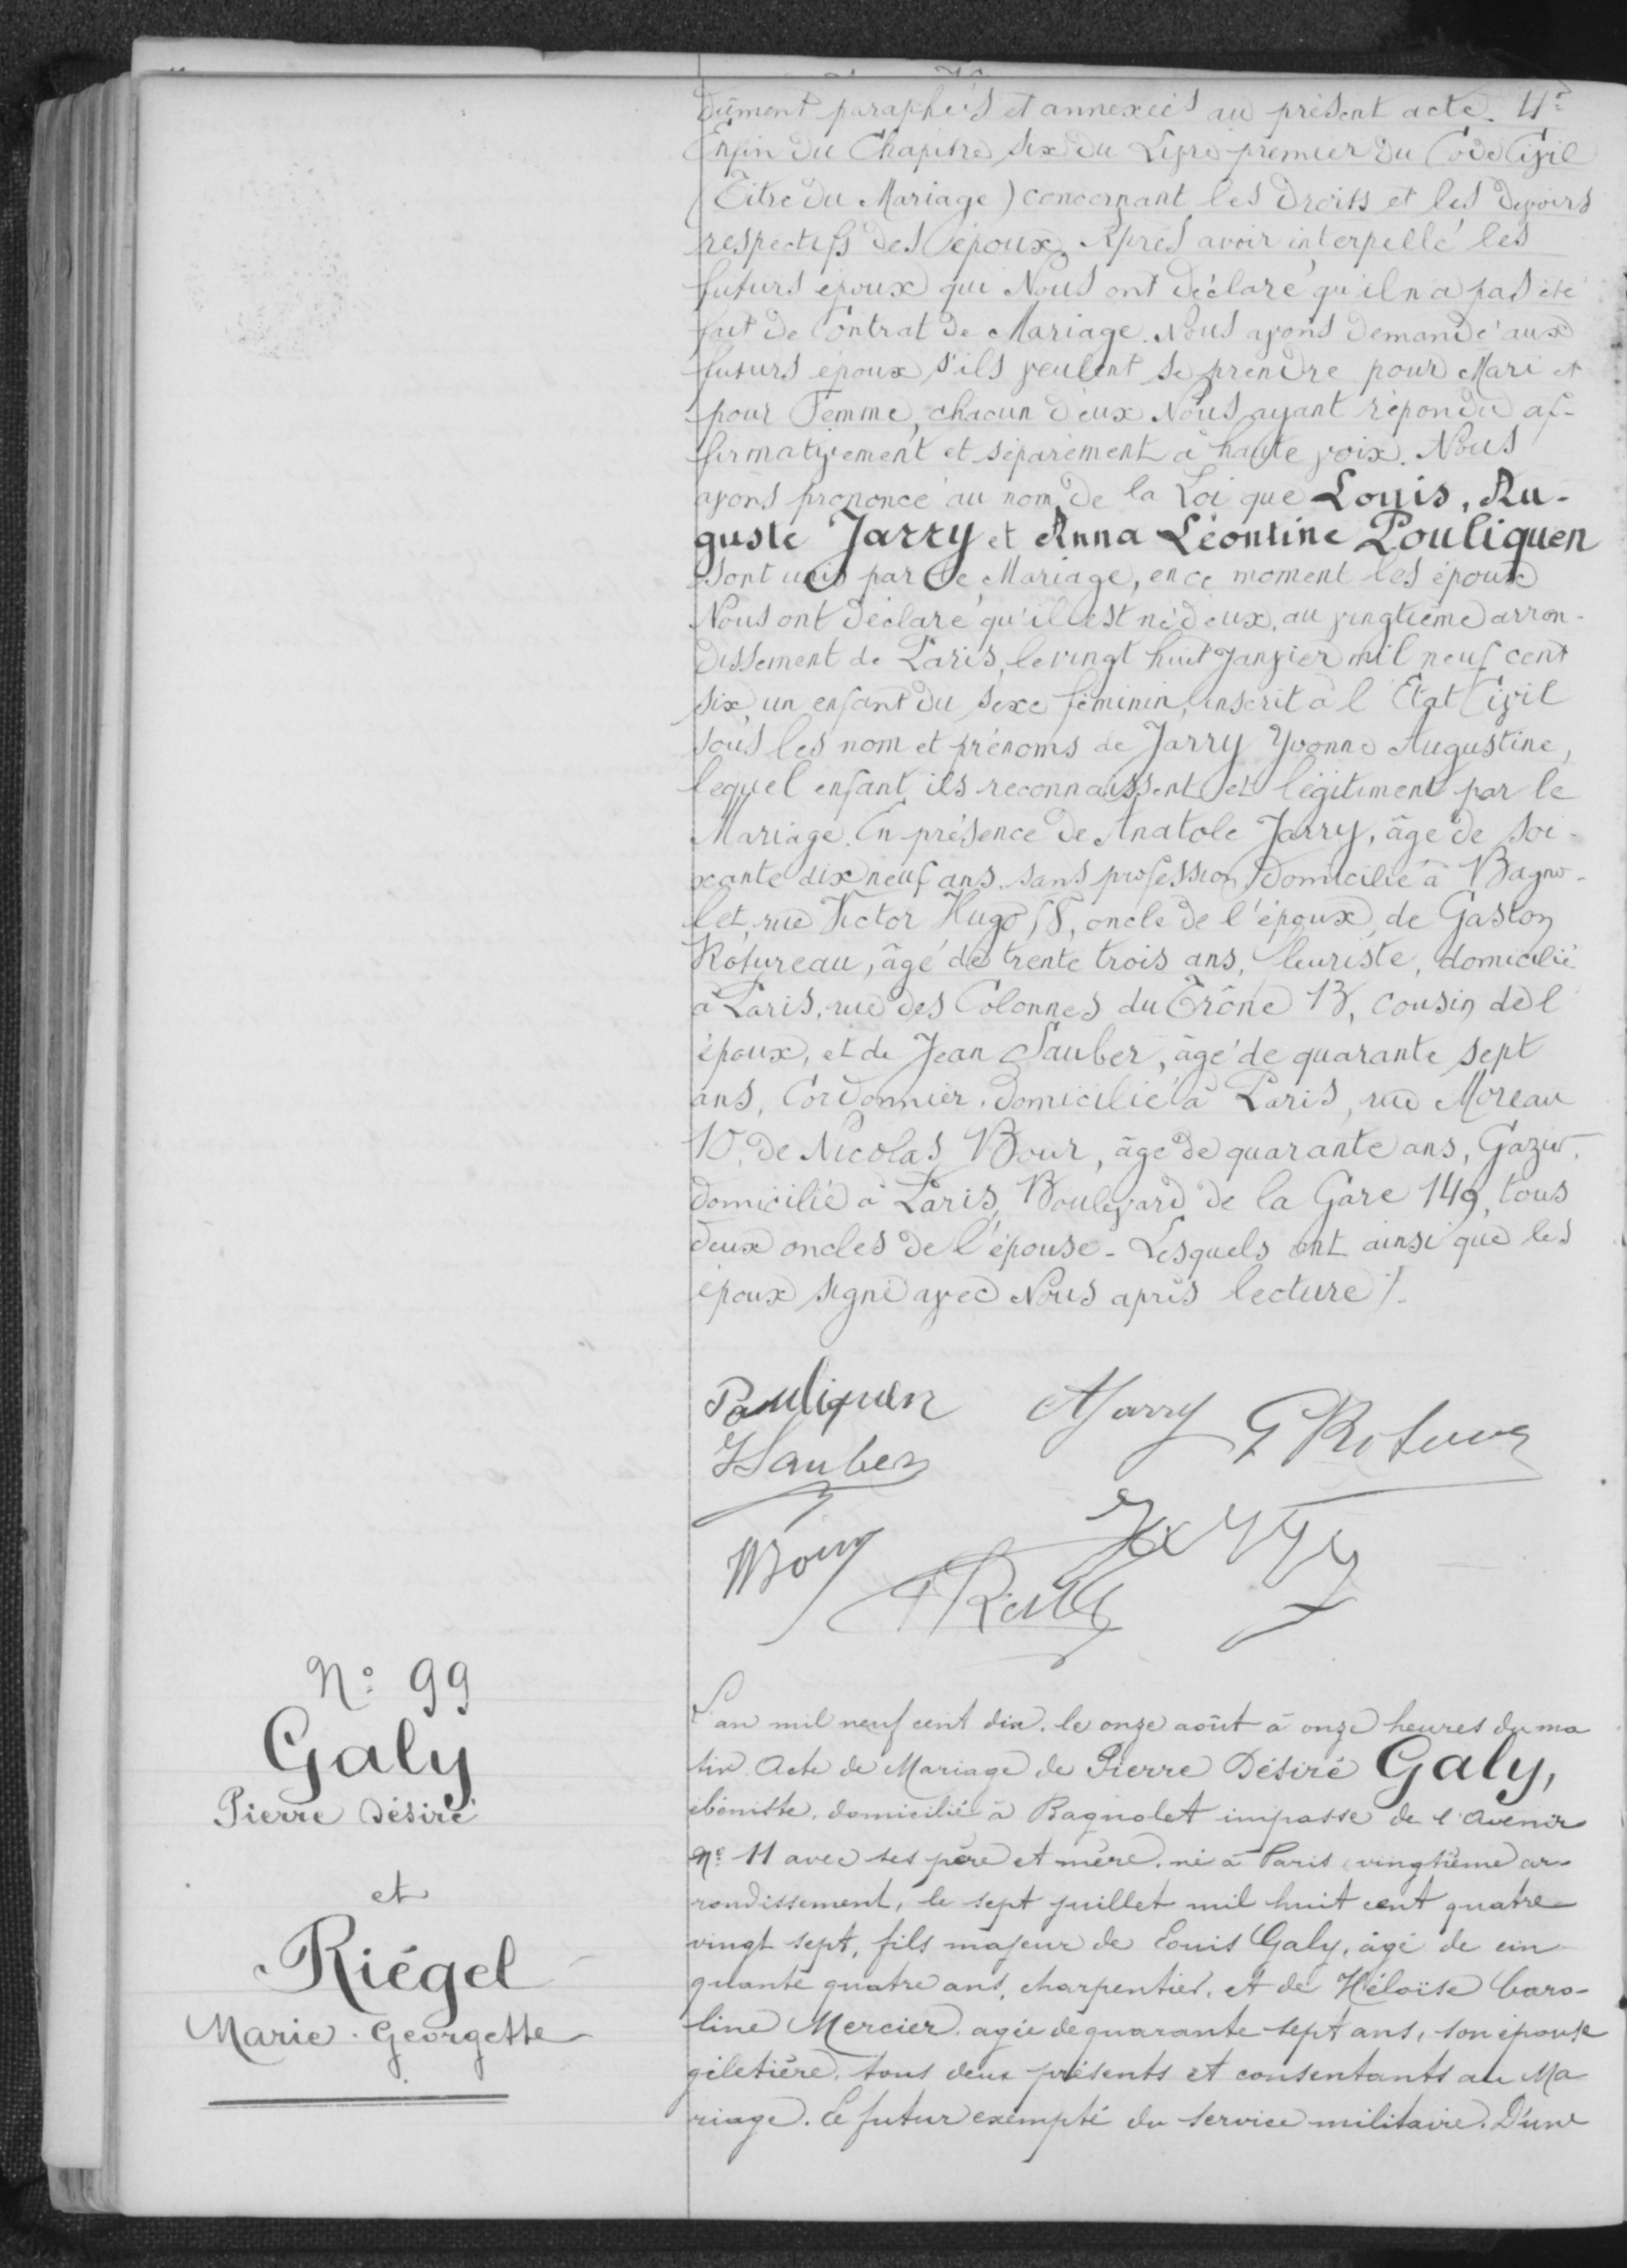dûment paraphées et annexées au présent acte. 4°
Enfin du Chapitre six du Livre premier du Code Civil
(Titre du Mariage) concernant les Droits et les devoirs
respectifs des époux. Après avoir interpellé les
futurs époux qui Nous ont déclaré qu'il n'a pas été
fait de contrat de Mariage. Nous ayons demandé aux
futurs époux s'ils veulent se prendre pour Mari et
pour Femme, chacun d'eux. Nous ayant répondu af-
firmativement et séparément à haute voix. Nous
avons prononcé au nom de la Loi que Louis, Au-
guste Jarry et Anna Léontine Pouliquen
sept unis par le Mariage, en ce moment les époux
Nous ont déclaré qu'il est né deux, au vingtième arron-
dissement de Paris, le vingt huit janvier mil neuf cent
six, un enfant du sexe féminin, inscrit à l'Etat Civil
sous les noms et prénoms de Jarry Yvonne Augustine,
lequel enfant ils reconnaissent et légitiment par le
Mariage. En présence de Anatole Jarry, âgé de soi
xante dix neuf ans sans profession domicilié à Bagno-
let, rue Victor Hugo 58, oncle de l'époux de Gaston
Rotureau, âgé de trente trois ans, fleuriste, domicilié
à Paris, rue des Colonnes du Trône 13, cousin de l'
époux, et de Jean Sauber, âgé de quarante sept
ans, cordonnier, domicilié à Paris, rue Morea
15 de Nicolas Bour, âgé de quarante ans, Gazur,
domicilié à Paris, Boulevard de la Gare 149, tous
deux oncles de l'épouse. Lesquels ont ainsi que les
époux signé avec Nous après lecture./.
N°99
Galy
Pierre Désiré
et
Riégel
Marie Georgette
L'an mil neuf cent dix le onze août à onze heures du ma
tin acte de Mariage, de Pierre Désiré Galy,
ébéniste, domicilié à Bagnolet impasse de l'avenir
N°:11 avec ses père et mère, né à Paris, vingtième ar-
rondissement, le sept juillet mil huit cent quatre
vingt sept, fils majeur de Louis Gaby, âgé de cin
quante quatre ans, charpentier, et de Héloïse Caro-
line Mercier, âgée de quarante sept ans, son épouse
géletière, tous deux présents et consentants au Ma
riage. Le futur exempté du service militaire. D'une
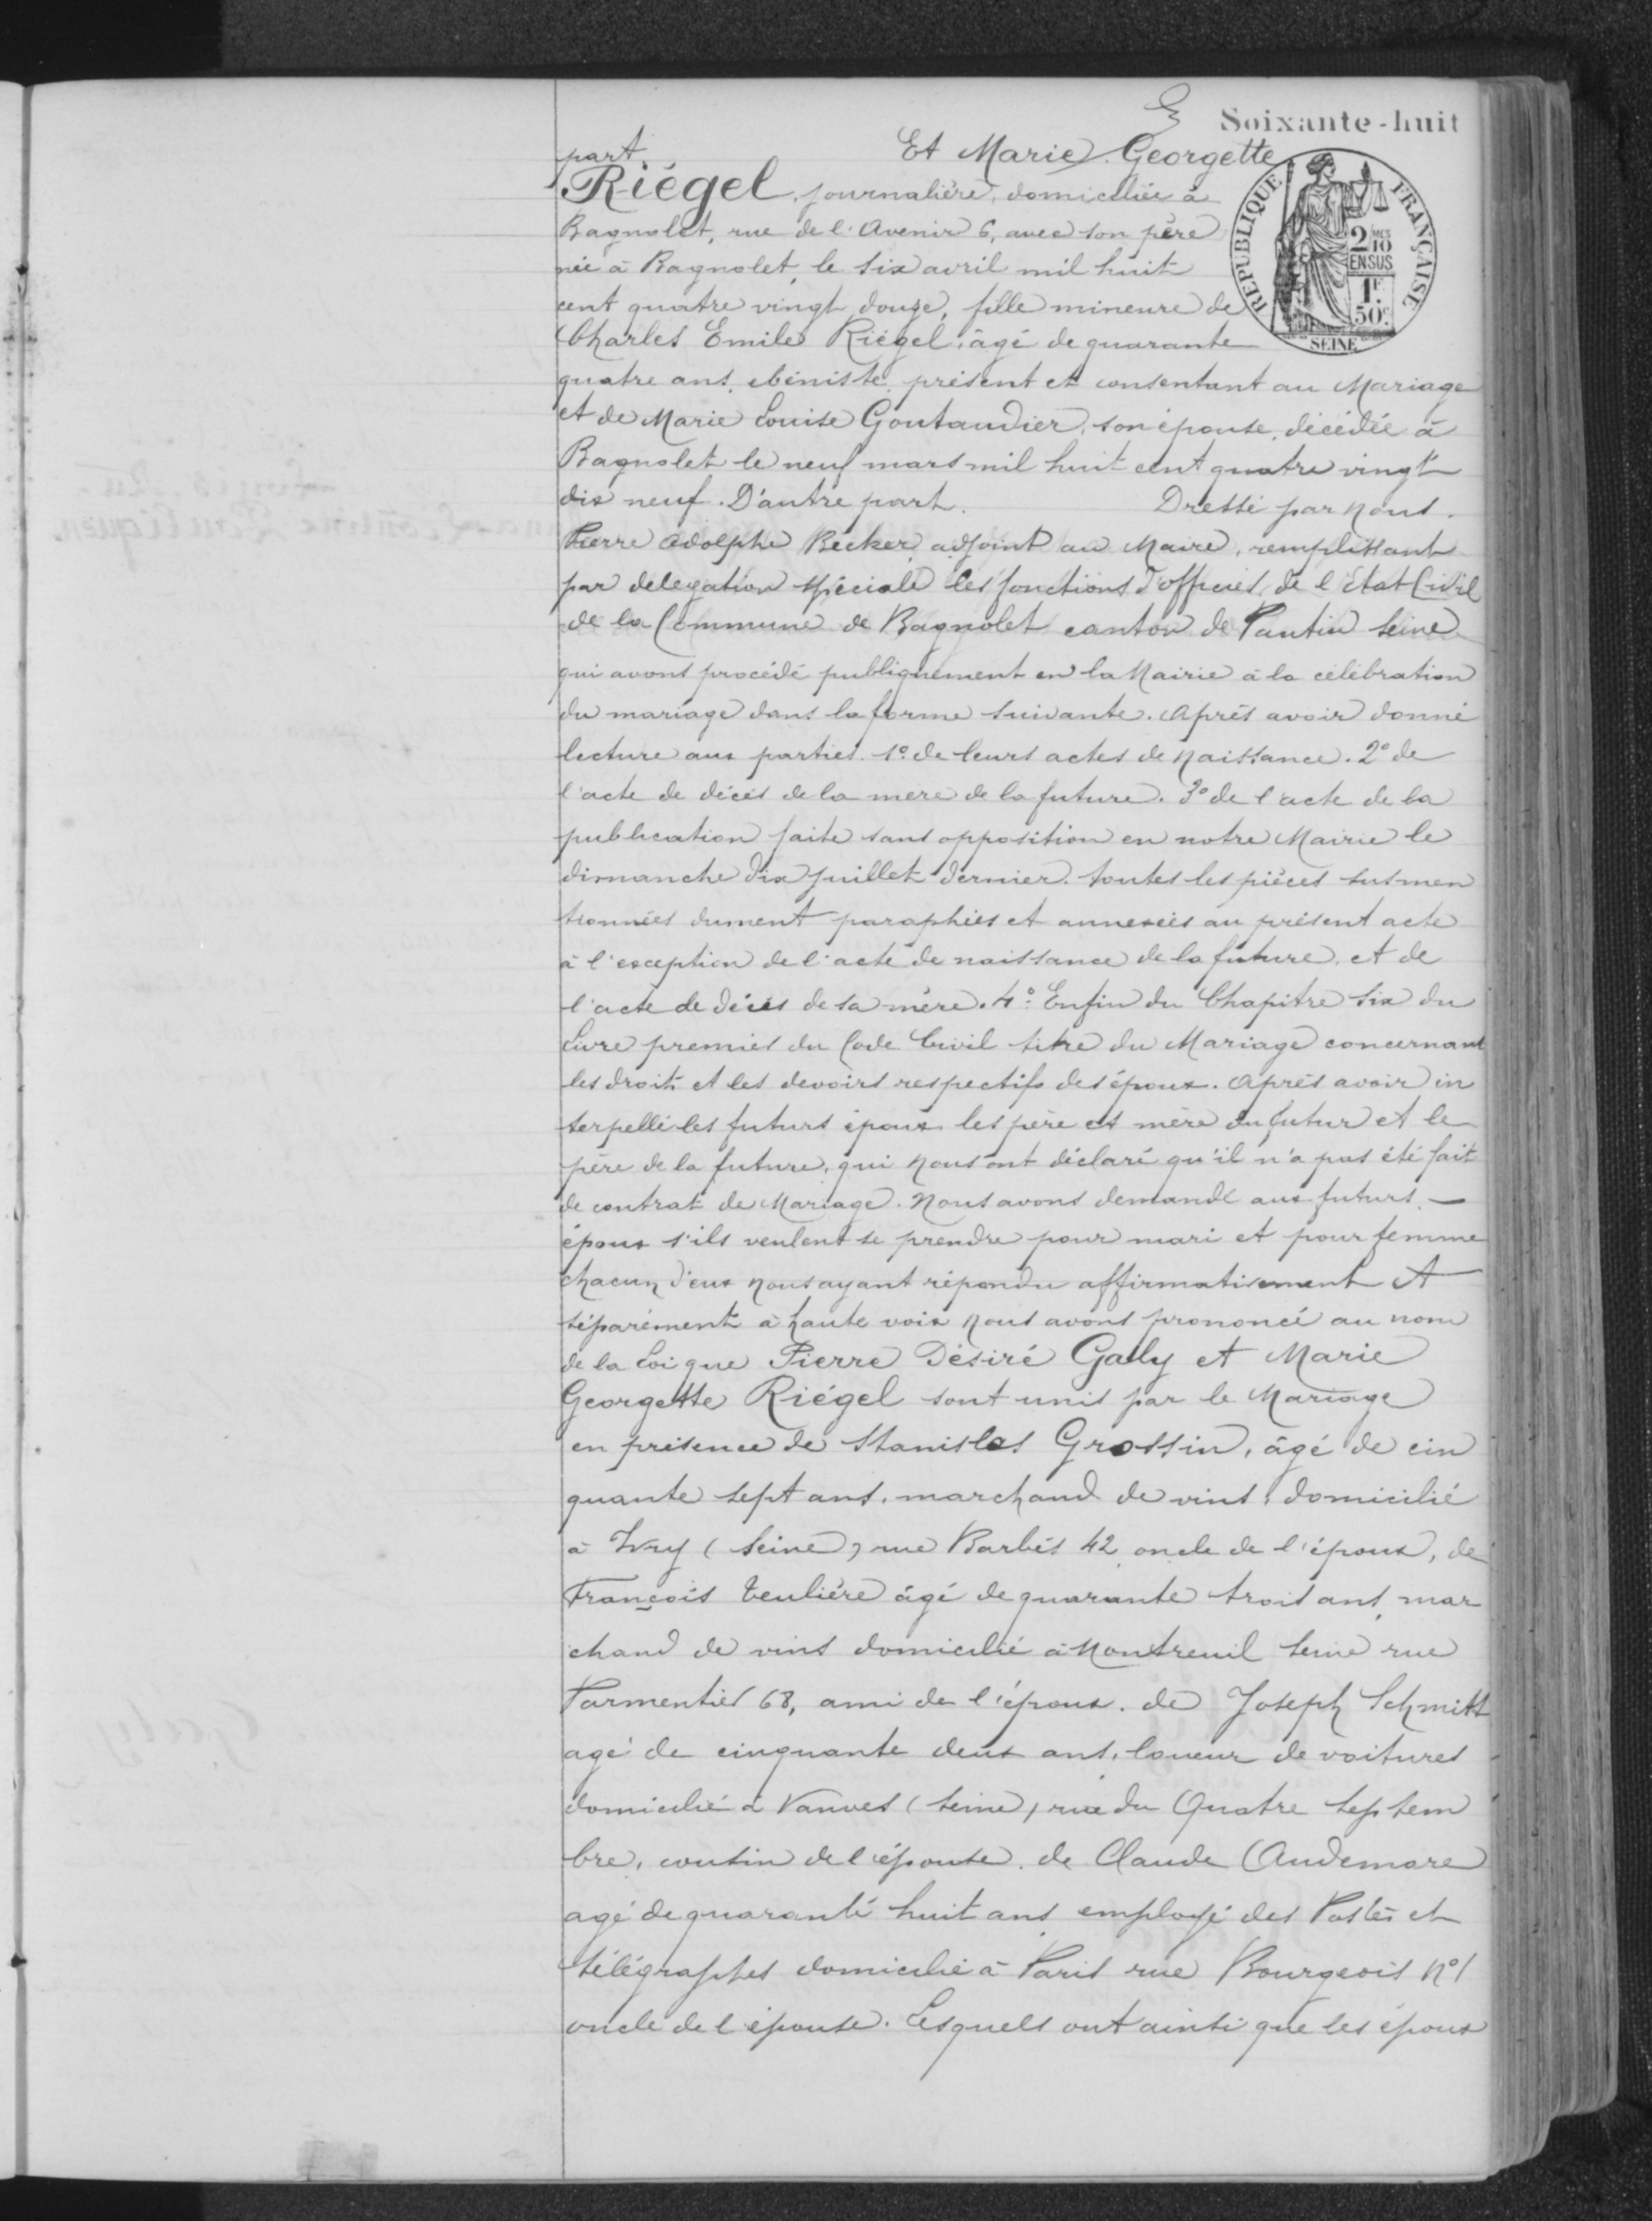part, Et Marie Georgette
Riégel, journalière, domiciliée à
Bagnolet, rue de l'Avenir 6, avec son père
née à Bagnolet, le six avril mil huit
cent quatre vingt douze, fille mineure de
Charles Emile Riégel, âgé de quarante
quatre ans, ebéniste, présent et consentant au Mariage
et de Marie Louise Goutaudier, son épouse, décédée à
Bagnolet le neuf mars mil huit cent quatre vingt
dix neuf. D'autre part. Dressé par nous
Pierre Adolphe Becker adjoint au Maire, remplissant
par delegation spéciale les fonctions d'officier de l'état Civil
de la commune de Bagnolet canton de Pantin Seine
qui avons procédé publiquement en la Mairie à la célébration
du mariage dans la forme suivante. Après avoir donné
lecture aux parties. 1° de leurs actes de naissance. 2° de
l'acte de décès de la mere de la future. 3° de l'acte de la
publication faite sans opposition en notre Mairie le
dimanche dix juillet dernier toutes les pièces susmen
tionnées dument paraphées et annexées au présent acte
à l'exception de l'acte de naissance de la future et de
l'acte de décès de la mère. 4°: Enfin du Chapitre six du
Livre premier du Code Civil titre du Mariage concernant
les droits et les devoirs respectifs des époux. après avoir in
terpellé les futurs époux les père et mère du futur et le
père de la future, qui nous ont déclaré qu'il n'a pas été fait
de contrat de Mariage. Nous avons demandé aux futurs
époux s'ils veulent se prendre pour mari et pour femme
chacun d'eux nous ayant répondu affirmativement et
séparément à haute voix nous avons prononcé au nom
de la Loi que Pierre Désiré Gaby et Marie
Georgette Riégel sont unis par le Mariage
en présence de Stanislas Grossin, âgé de cin
quante sept ans, marchand de vins domicilié
à Ivry (Seine) rue Barbès 42 oncle de l'épouse, de
François Zeubière âgé de quarante trois ans, mar
chand de vins domicilié à Montreuil seine rue
Parmentier 68, ami de l'épouse, de Joseph Schmitt
agé de cinquante deux ans, loueur de voitures
domicilié à Vannes (Seine), rue du Quatre Septem
bre, cousin de l'épouse, de Claude Andemare
agé de quarante huit ans employé des Postes et
télégraphes domicilié à Paris rue Bourgeois n°1
oncle de l'épouse. Lesquels ont ainsi que les époux